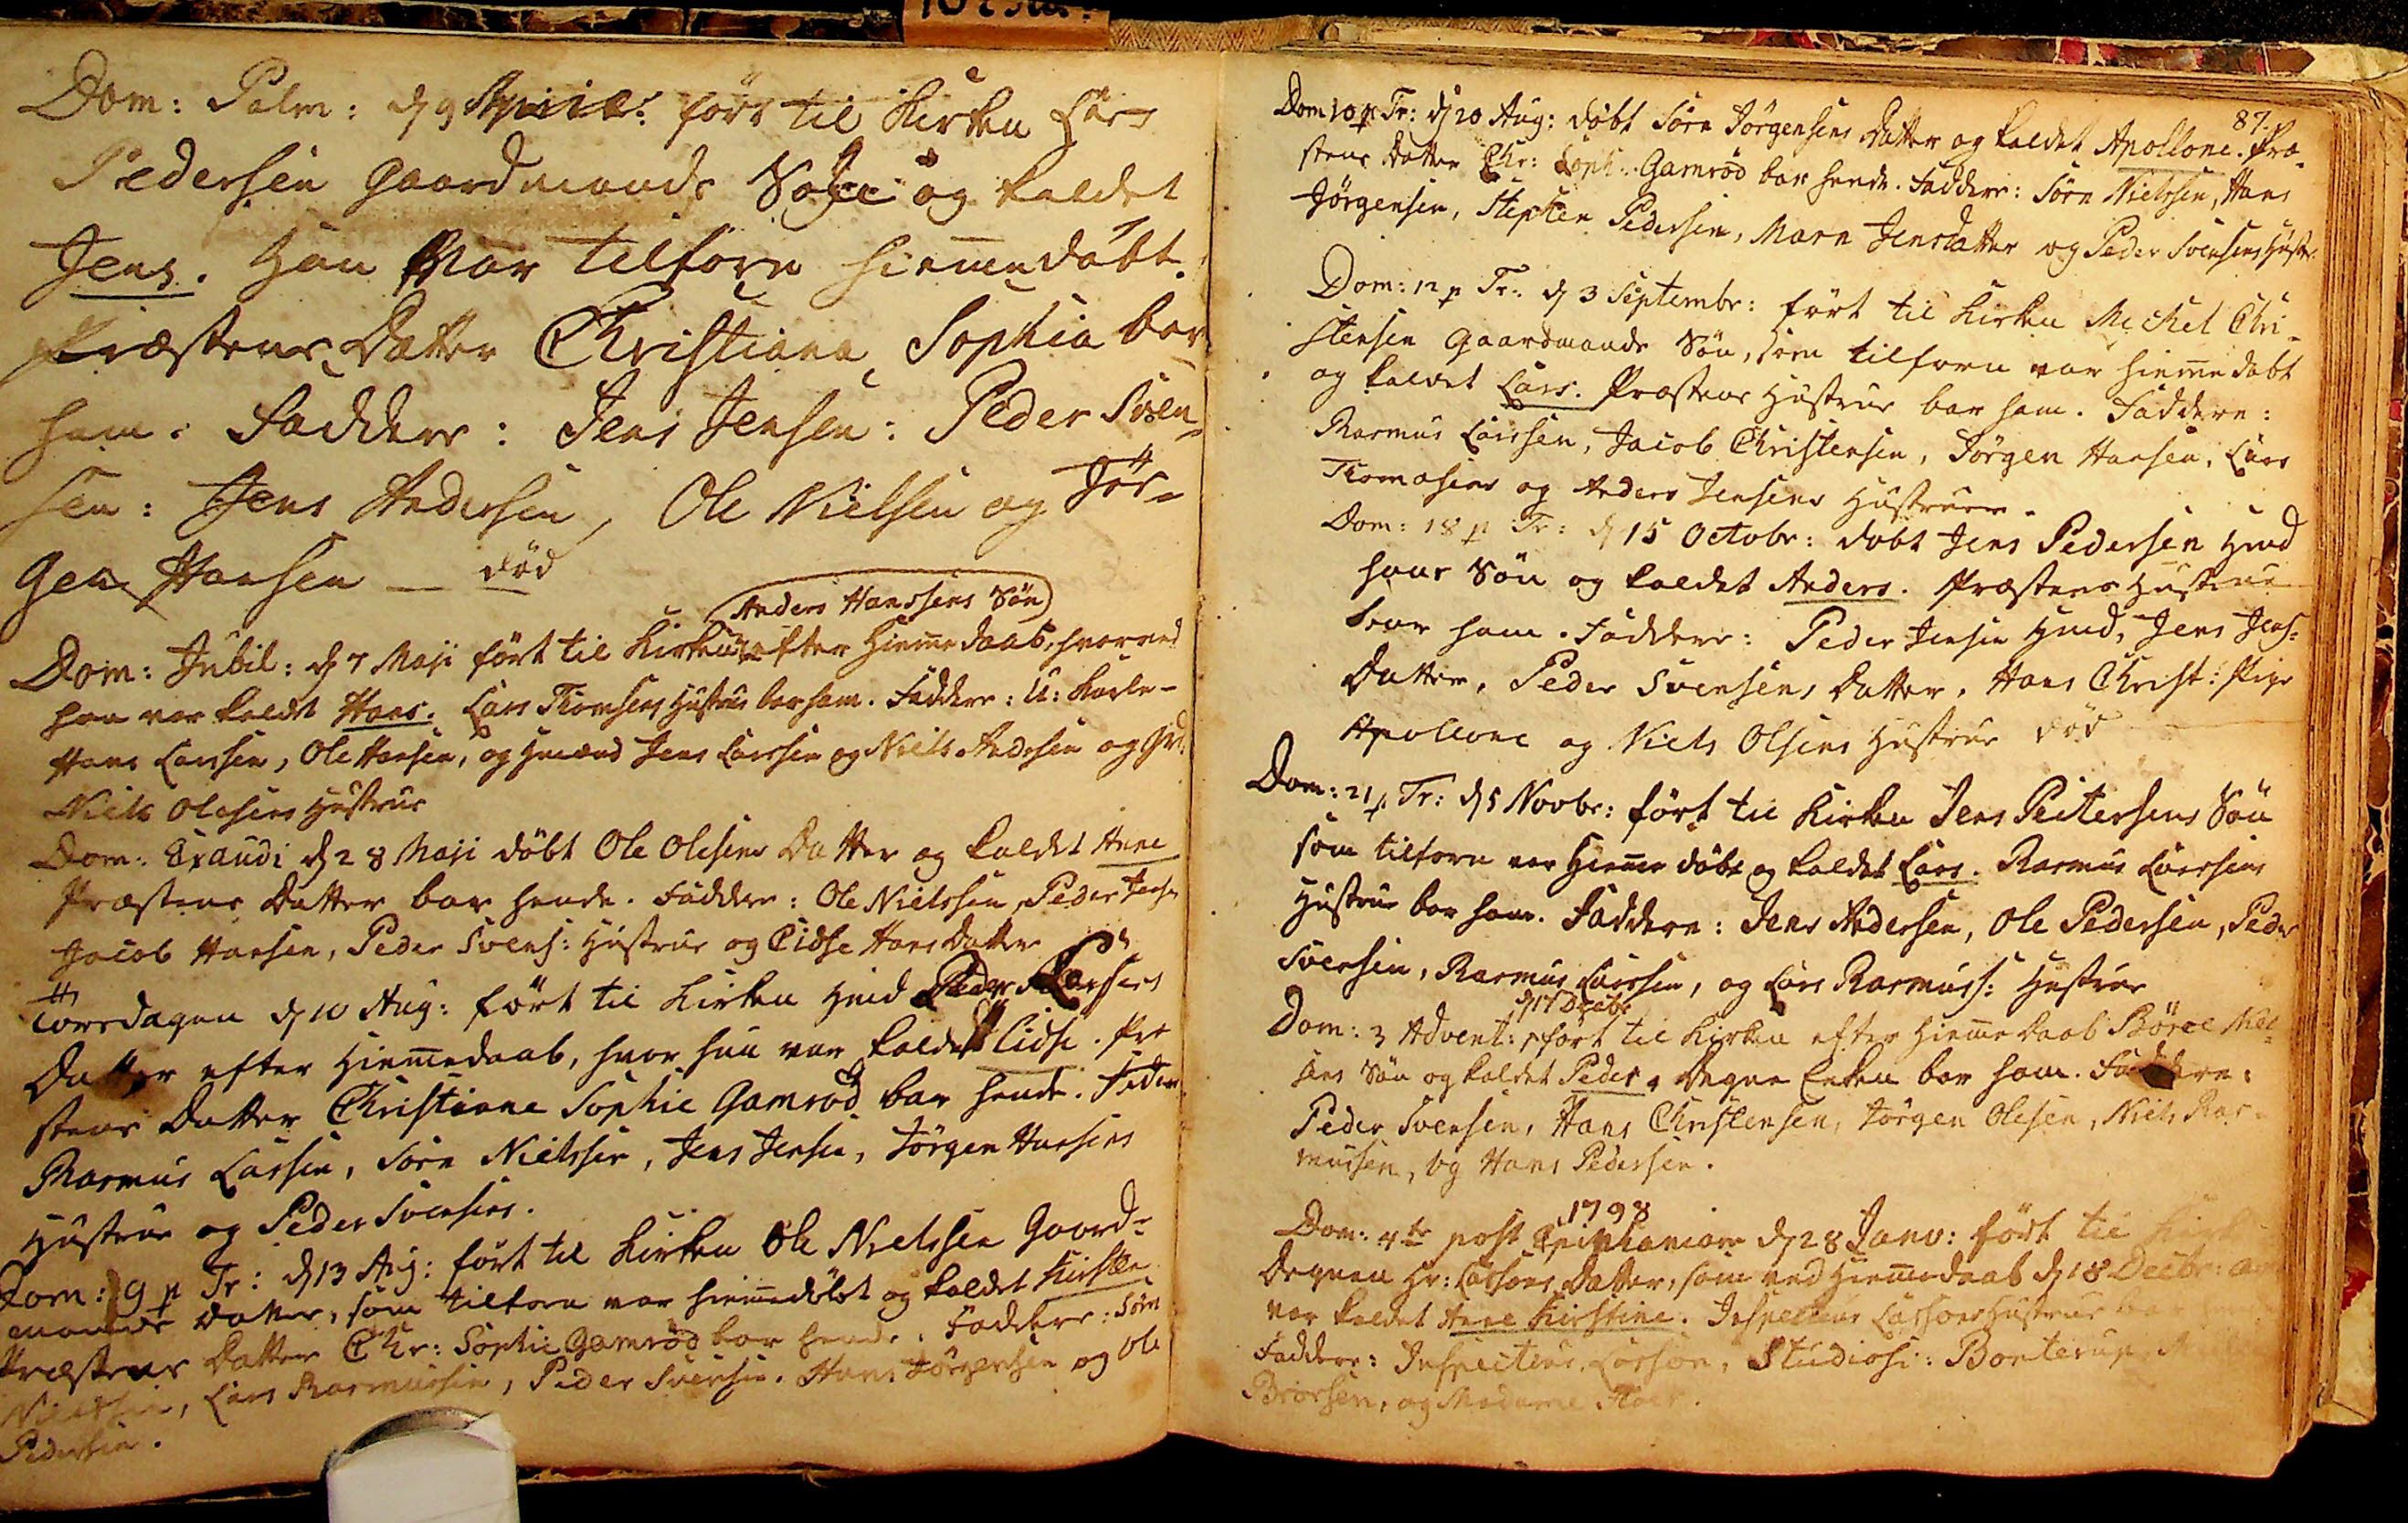Dom: Palm. d 9 April: ført til Kirken Lars
Pedersen Gaardmands Søn og kaldet
Jens. Han var tilforn hiemmedøbt.
Præstens Datter Christiana Sophia bar
ham. Faddere: Jens Jensen: Peder Sven¬
sen: Jens Andersen, Ole Nielsen og Jør¬
gen Hansen
død
Anders Hansens Søn
Dom: Jubil: d 7 Maji ført til Kirken efter hiemmedaab: hvorved
han var kaldt Hans. Lars Thomsens Hustru bar ham. Faddere: U: Karle -
Hans Larssen, Ole Hansen, og Hmænd Jens Larssen og Niels Andersen og Grd:
Niels Olesens Hustrue
Dom: Exaudi d 28 Maji døbt Ole Olesens Datter og kaldet Anne
Præstens Datter bar hende. Faddere: Ole Nielssen, Peder Jensen
Jacob Hansen, Peder Svens: Hustrue og Cidse Hans Datter
Torsdagen d 10 Aug: ført til Kirken Hmd. Peder Larsens
Datter efter Hiemmedaab, hvor hun var kaldet Cidse. Præ
stens Datter Christiane Sophie Gamrød bar hende. Fadere
Rasmus Larsen, Sørn Nielssen, Jens Jensen, Jørgen Hansens
Hustrue og Peder Svensens.
Dom: 9 p Tr: d 13 Aug: ført til Kirken Ole Nielssen Gaard¬
mands Datter, som tilforn var hiemmedøbt og kaldet Kirsten
Præstens Datter Chr: Sophie Gamrød bar hende. Faddere: Sørn
Nielsen, Lars Rasmussen, Peder Svensen. Hans Jørgensen og Ole
Pedersen.
87.
Dom:10 p Tr: d 20 Aug: døbt Sørn Jørgensens Datter og kaldet Apollone. Præ¬
stens Datter Chr: Soph: Gamrød bar hende. Faddere: Sørn Nielssen, Hans
Jørgensen, Stephen Pedersen, Marn Jensdatter og Peder Svensens Hustr.
Dom: 12 p Tr: d 3 Septembr: ført til Kirken Michel Chri¬
stensen Gaardmands Søn, som tilforn var hiemmedøbt
og kaldet Lars. Præstens Hustrue bar ham. Faddere:
Rasmus Larssen, Jacob Christensen, Jørgen Hansen, Lars
Thomæsens og Anders Jensens Hustruer.
Dom: 18 p Tr: d 15 Octobr: døbt Jens Pedersen Hmd
hans Søn og kaldet Anders. Præstens Hustrue
bar ham. Faddere: Peder Jensen Hmd, Jens Jens¬
Datter, Peder Svensens Datter, Hans Christ: Pige
Apollone og Niels Olsens Hustrue død.
Dom: 21 p Tr: d 5 Novbr: ført til Kirken Jens Peitersens Søn
som tilforn ere Hiemme døbt og kaldet Lars. Rasmus Larsens
Hustrue bar ham. Faddere: Jens Andersen, Ole Pedersen, Peder
Svensen, Rasmus Larssen, og Lars Rasmuss: Hustrue
d 17 Decbr
Dom: 3 Advent: ført til Kirken efter Hiemme Daab Børre Niel¬
sens Søn og kaldet Peder. Degne Enken bar ham. Faddere:
Peder Svensen, Hans Christensen, Jørgen Olesen, Niels Ras¬
mussen, og Hans Pedersen.
1798
Dom: 4te post Epiphaniam d 28 Janv: ført til Kirke
Degnen Hr: Larsons Datter, som ved Hiemmedaab d 18 Decbr: A:
var kaldet Anne Kirstine. Inspecteur Lassons Hustrue bar hende
Faddere: Inspecteur Lasson, Studiosi: Bo#terup, Müller
Brorsen, og Madame ##.